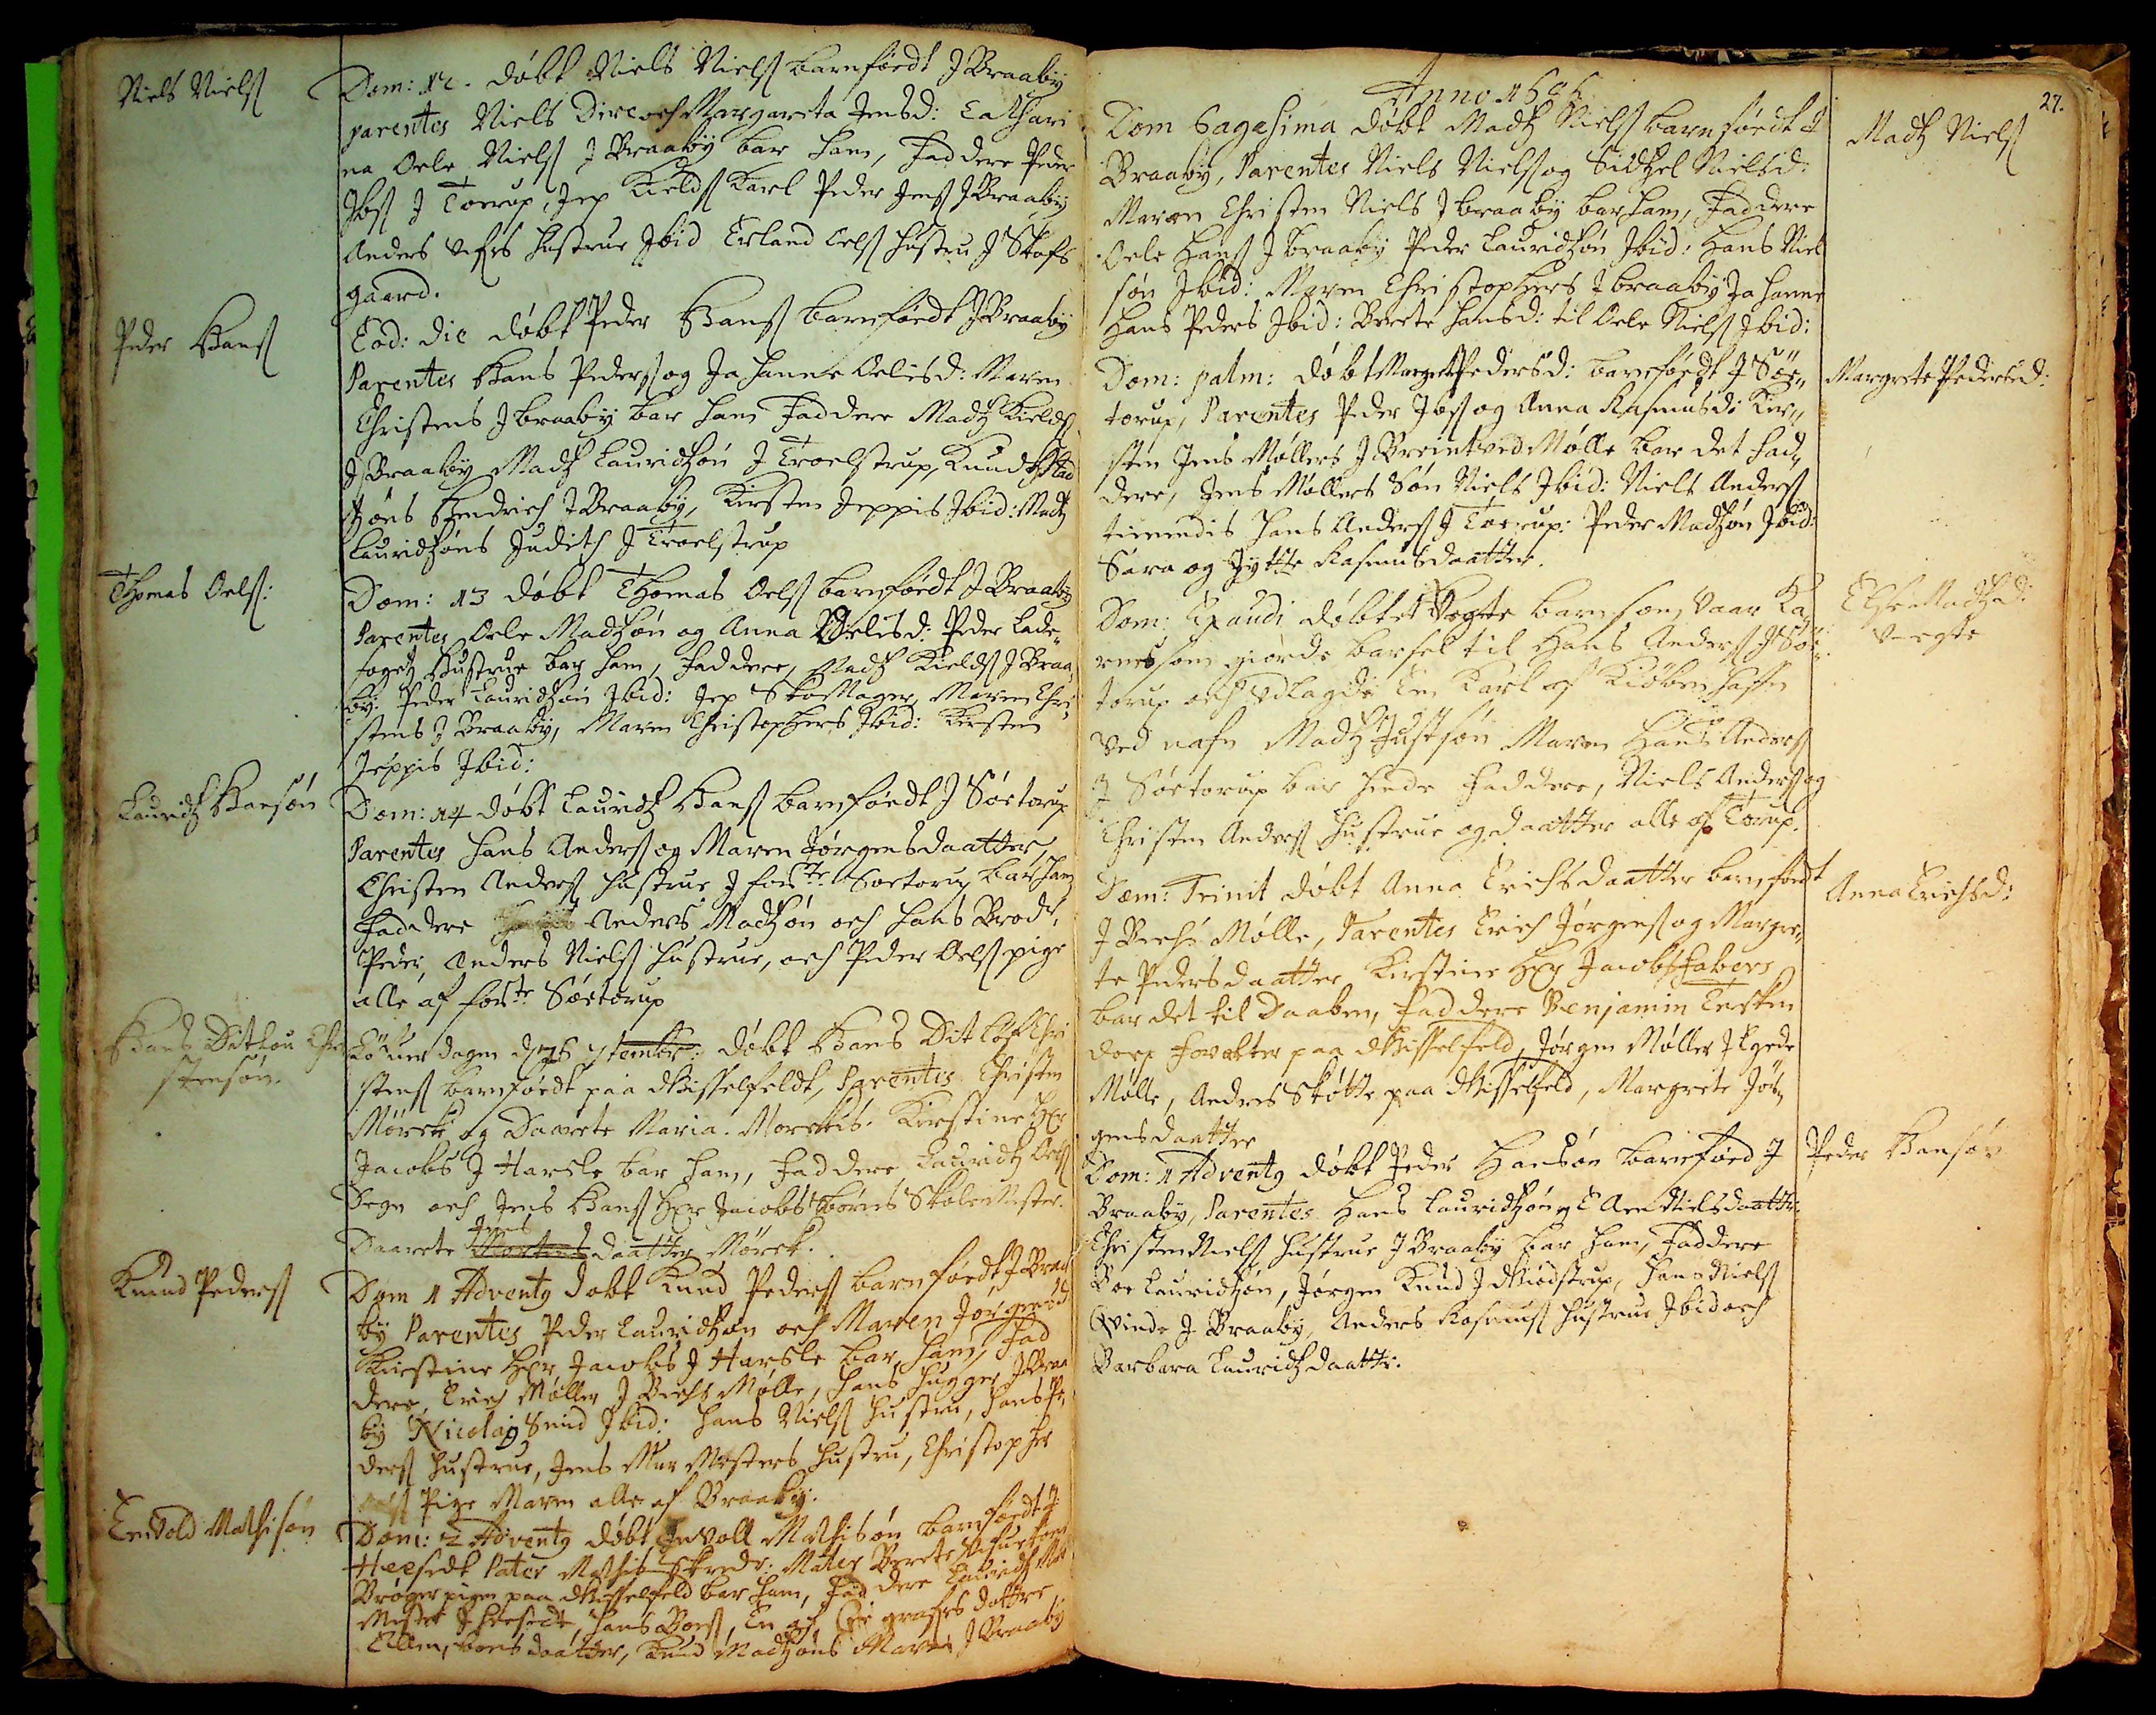Niels Niels
Dom: 12. døbt Niels Niels barnføedt J Braaby
Parentes Niels Dire och Margereta Jensd: Catheri
na Oele Niels J Braaby bar ham, Faddere Peder
Jbs J Toerup, Jep Kields karl Peder Jens J Braaby
Anders Vefis Hustrue Jbid, Erland Oels Hustru J Skofs
gaard.
Peder Hans
Eod: die døbt Peder Hanss barn føedt J Braaby
Parentes Hans Peders og Johanne Oelisd: Maren
Christens J Braaby, bar ham Faddere Mads Kields
J Braaby Mads Lauridsøn J Troelstrup, Knud Oele##
søns Hendrich J Braaby, Kirsten Jeppis Jbid: Mads
Lauridsøns Judith J Troelstrup.
Thomas Oels:
Dom: 13 døbt Thomas Oels barnføedt J Braaby
Parentes Oele Madsøn og Anna Oelisd: Peder Lade¬
fogetz Hustrue bar ham, Faddere Mads Kields J Braa¬
by: Peder Laurissøn Jbid: Jep SkoeMager, Maren Chri¬
stens J Braaby, Maren Christophers Jbid: Kirsten 
Jeppis Jbid: 
Lauridz Hansøn
Dom: 14 Lauridz Hans barn føedt J Søetorup,
Parentes Hans Anders og Maren Jørgensdaatter,
Christen Anders Hustrue J forte Søetorup bar ham.
Faddere: Hans Anders Madsøn och Hans Broder
Peder Anders Niels hustrue, och Peder Oels pige
alle af forte Søetorup.
Hans Ditleu Chri
stensøn
Løfuerdagen d 26 7tembr døbt Hans Ditløf Chri
stens barnføedt paa Gisselfeldt, Parentes: Christen
Mørck og Daarete Maria Mørckis Kirstine Hxr
Jacobs J Harsle bar ham, Faddere Laurids Oels
Degn och Jens Hans Hxr Jacobs børns SkoleMester,
Jens
Daarete Mortens daatter Mørck.
Knud Peders
Dom 1 Adventy døbt Knud Peders barn føedt J Braa
by, Parentes: Peder Lauridsøn och Maren Jørgensd:
Kirstene Hxr Jacobs J Harsle bar ham, Fad
dere, Erich Møller J Bechs Mølle, Hans Hugger J Braa
by, Nicolay Smid Jbid: Hans Niels Hustrue, Hans Pe¬
ders Hustrue, Jens MureMesters Hustrue, Christopher
Oels## Pige Maren alle af Braaby:
Envold Mathisøn
Dom: 2 Adventy døbt Envold Mathisøn barnføedt J
Heesedt, Pater Mathis Skreder: Mater Berete Vefur, ##
Brøger pige paa Gisselfeld bar ham, Faddere Laurids ##
Mester J Heesedt, Hans Boes, En af Cei grafzes dattre
Ellen Ceisdatter, Knud Madsøns Maren J Braaby.
Anno 1686.
Dom 6agesima døbt Madz Niels barn føedt J
Braaby, Parentes Niels Niels og Sidzel Nielsd:
Maren Christen Niels J Braaby bar ham, Faddere
Oele Hans J Braaby, Peder Lauridzøn Jbid. Hans Niel
søn Jbid: Maren Christophers J Braaby, Johanne
Hans Peders Jbid: Borete Hansd: til Oele Niels Jbid:
27.
Madz Niels
Dom: palm: døbt Margrete Pedersd: barnføedt J Søe¬
torup, Parentes Peder Jbs og Anna Rasmusd: Kir¬
sten Jens Møllers i Breintved Mølle bar det Fad¬
dere, Jens Møllers Søn Niels Jbid: Niels Anders
tienendis Hans Anders J Toerup: Peder Madsøn Jbid:
Sara og Jytte Rasmusdaatter. 
Margrete Pedersd:
Dom: Exaudi døbt et Uegte barn som vaar Ka¬
rens som giorde barsel til Hans Anders J Søe¬
torup och udlagde En Karl af Kiøbenhaffen
ved nafn Madz Justsøn, Maren Hans Anders
J Søetorup bar hinde Faddere, Niels Anders og
Christen Anders Hustrue og daatter alle af Toerup.
Else Madzd: 
U-egte
Dom: Trinit døbt Anna Erichsdatter barnføedt
J Bechs Mølle, Parentes Erich Jørgens og Margre¬
te Pedersdaatter, Kirsten Hxr Jacobs Fabers
bar det til Daaben, Faddere Benjamin Tesken##
dorp## forvalter paa Gisselfeld, Jørgen Møller J Egede
Mølle, Anders Skøtte paa Gisselfeld, Margrete Jør¬
gensdaatter.
Anna Erichsd:
Dom: 1 Adventy døbt Peder Hansøn barneføed J
Braaby, Parentes Hans Lauridsøn og Ellen Nielsdaatter,
Christen Niels Hustrue J Braaby bar ham, Faddere
Boe Lauridsøn, Jørgen Knud J Giødstrup, Hans Niels
Qvinde J Braaby, Anders Rasmus Hustrue Jbid. och
Barbara Lauridsdaatter.
Peder Hansøn
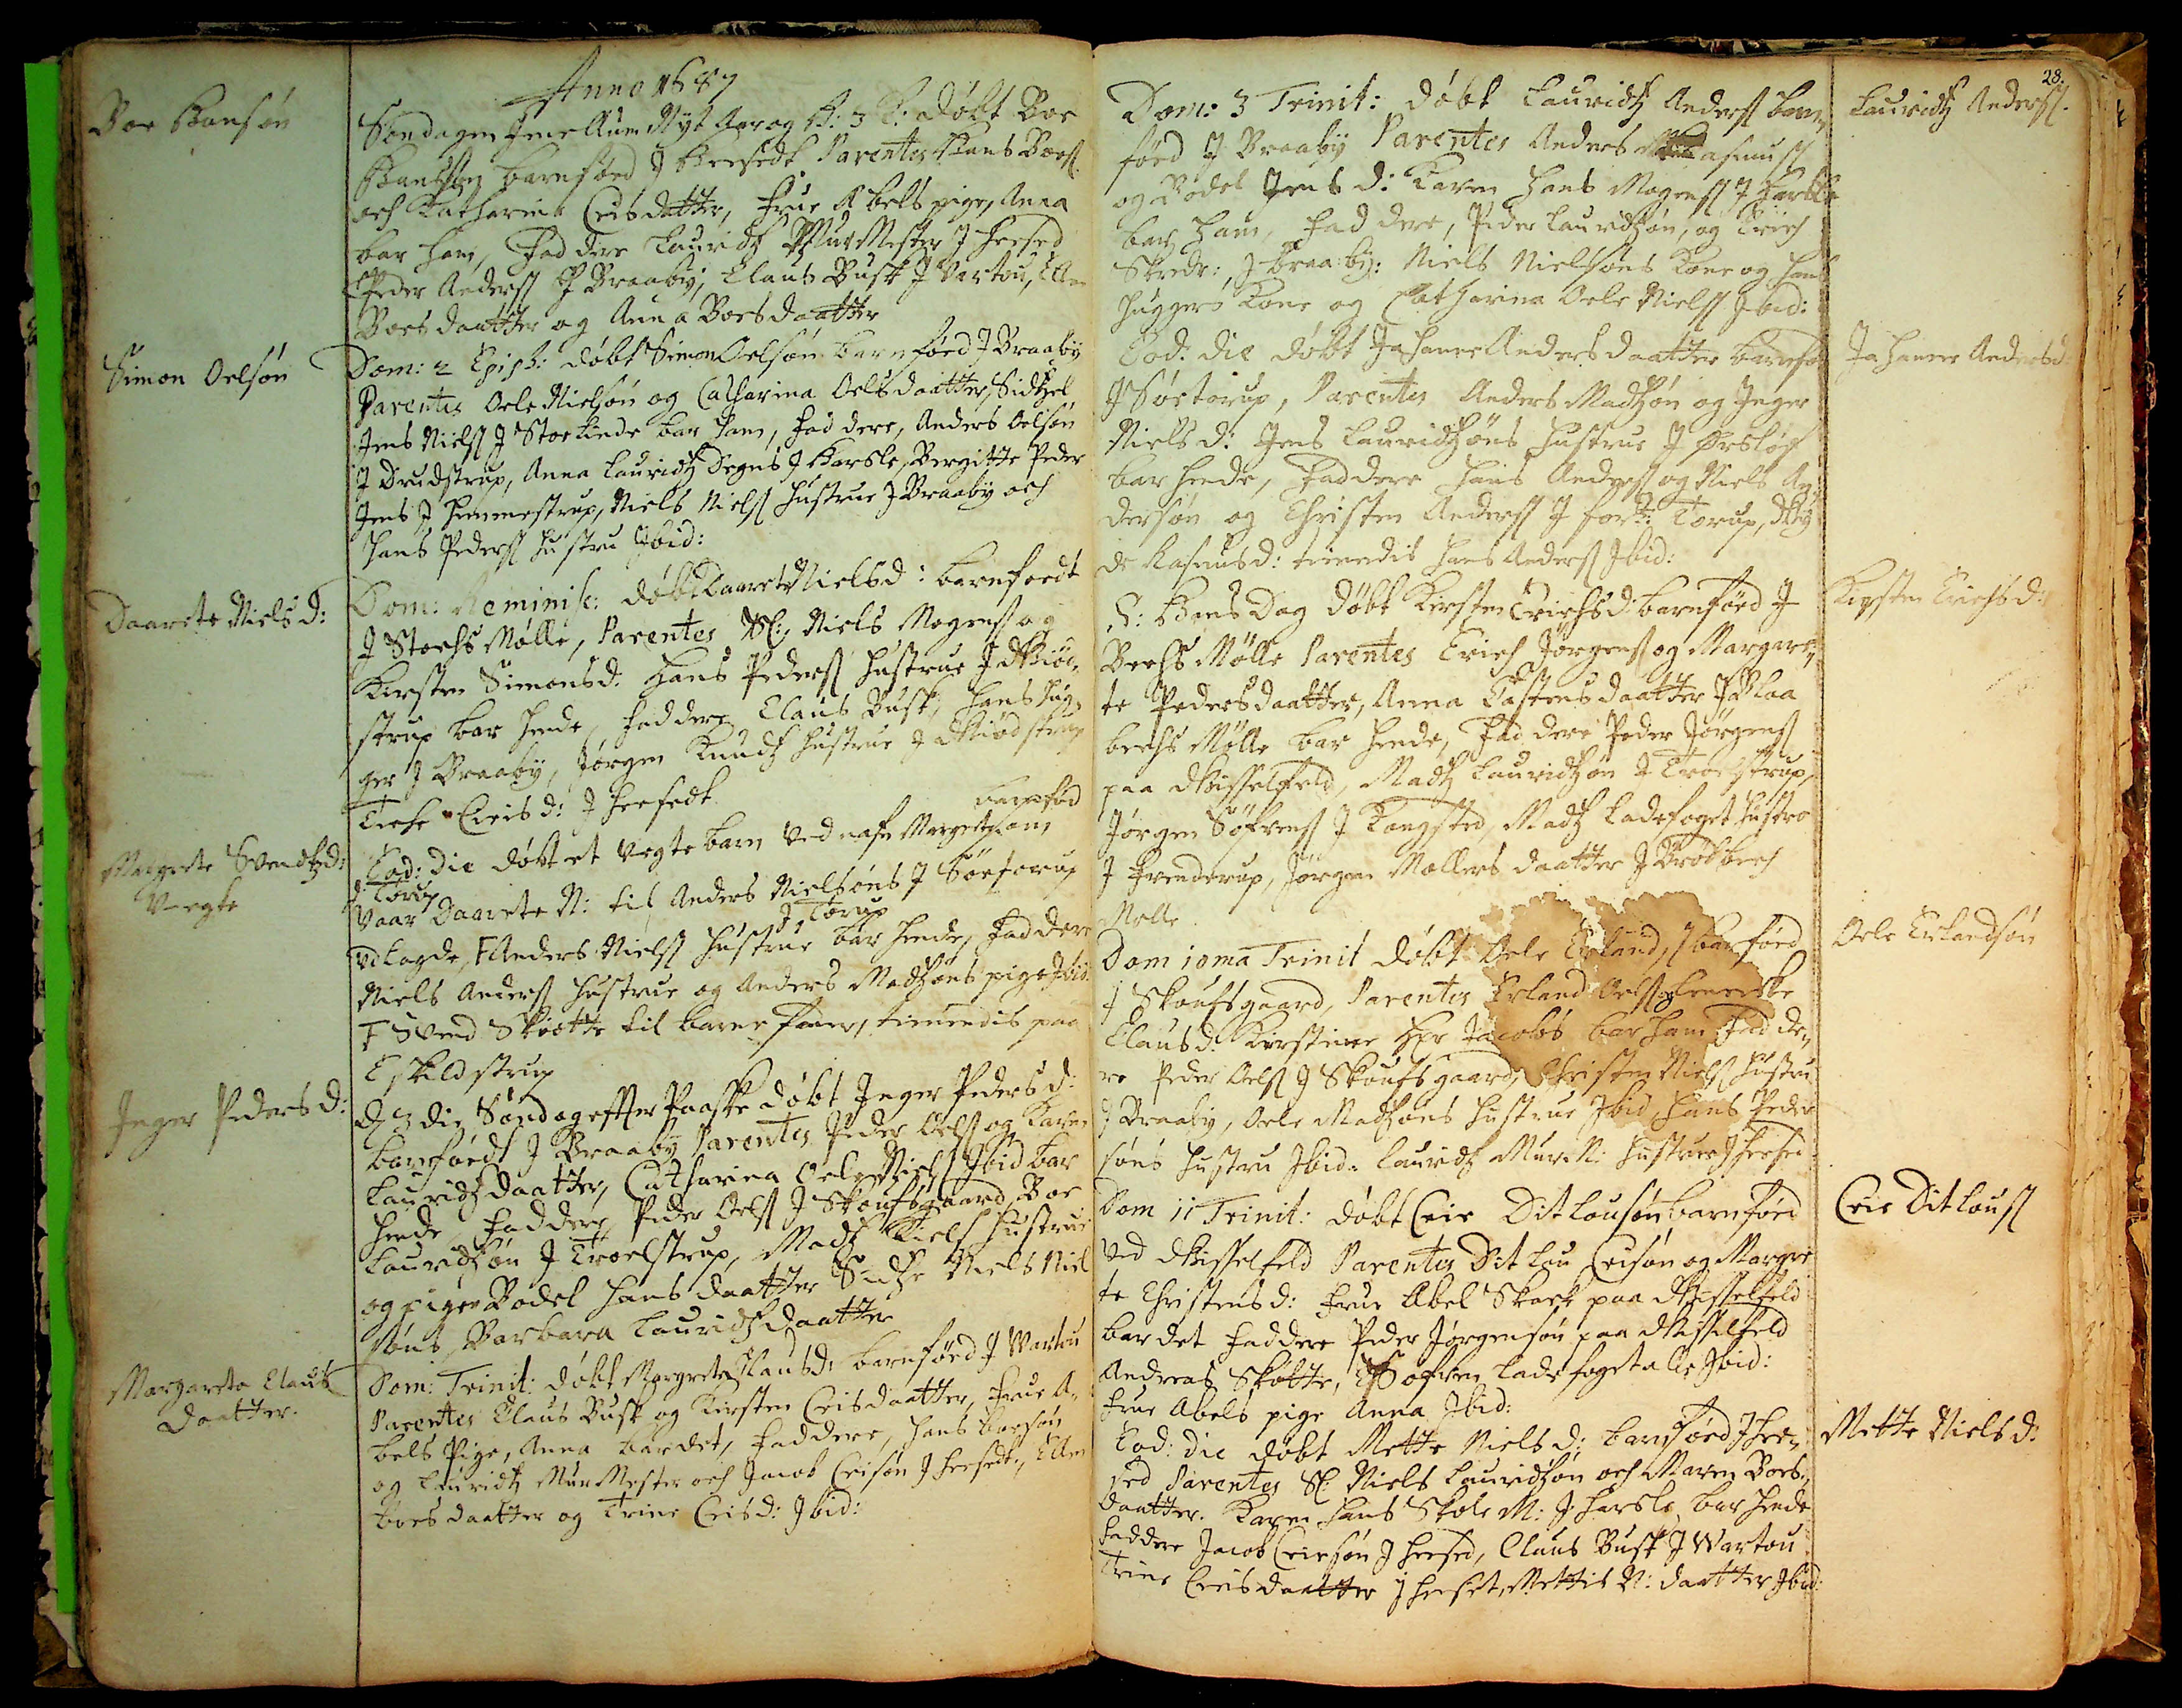Boe Hansøn 
Anno 1687 
Søndagen Imellum Nyt Aar og H: 3 K: døbt Boe 
Hansøn barnføed J Heesedt Parentes Hans Boes
och Katharine Ceisdatter, Frue Abels pige, Anna
bar ham, Faddere Laurids MurMester i Heesed
Peder Anders J Braaby; Claus Busk i Vartou, Ellen
Boes daatter og Anna Boes daatter.
Simon Oelsøn 
Dom: 2 Epiph døbt Simon Oelsøn barnføed J Braaby 
Parentes Oele Nielsøn og Catharina Oelsdaatter, Sidsel
Jens Niels J Stor Linde bar ham, Faddere, Anders Oelsøn
J Drudstrup, Anna Laurids Degns J Harsle, Bergitte Peder
Jens J Hemmestrup, Niels Niels Hustrue J Braaby och
Hans Peders Hustru Ibid:
Daarete Niels d: 
Dom: Reminisc: døbt Daarete Nielsd: barneføedt 
J Stoechs Mølle, Parentes Sl: Niels Mogens og
Kirsten Simonsd. Hans Peders Hustrue J Giød¬
strup bar hende, Faddere Claus Busk, Hans Hug¬
ger J Braaby, Jørgen Knuds Hustrue J Giødstrup
Tiehne## Ceis d: J Heesedt.
Margrete Svendsd:
Ueegte
barnefød
Eod: die døbt et Uegte barn ved nafn Margrete som
J Torup, 
vaar Daarete N: til Anders Nielsøn J Søetorup 
J Torup
udlagde F. Anders. Niels Hustrue bar hende, Fadder
Niels Anders Hustrue og Anders Madsøns pige Jbid
F. Svend Skiøtte til barne Fader, tienendis paa
Eskildstrup.
Jnger Peders d: 
D 3dig Søndag efter Paaske døbt Jnger Peders d: 
barneføedt J Braaby Parentes Peder Oels og Karen
Lauridsdaatter, Cathrina Oele Niels Jbid bar
hende, Faddere Peder Oels J Skoufsgaard, Boe
Lauridsøn J Troelstrup, Mads Kiels Hustrue
og piger Bodel Hans daatter, Sidse Niels Niel
søns, Barbara Lauridsdaatter.
Margrete Claus 
Daatter.
Dom: Trinit: døbt Margrete Clausd: barnføed J Vartou 
Parentes Claus Busk og Kirsten Ceisdaatter, Frue A¬
bels Pige, Anna bar det, Faddere, Hans Boesøn 
og Laurids, MurMester och Jacob Ceisøn J Heesedt, Ellen
Boes datter og Trine Ceisd: Jbid:
Dom: 3 Trinit: døbt Laurids Anders barn¬
føed J Braaby Parentes Anders Rasmus 
og Bodel Jensd: Karen Hans Mogens J Harsle
bar ham, Faddere, Peder Lauridsøn, og Erich
Skredr: J Braaby: Niels Nielsøns Kone og Hans
Huggers kone og Catherina Oele Niels Jbid:
28.
Lauridz Anders.
Eod: die døbt Johanne Andersdaatter barnefød 
J Søetorup, Parentes Anders Madsøn og Jnger
Nielsd:. Jens Lauridsøns Hustrue i Ørsløf
bar hende, Faddere Hans Anders og Niels An¬
dersøn og Christen Anders J Forte. Torup, Gy
de Rasmusd: tienndis Hans Anders Jbid:
Johanne Andersd: 
S: Hans Dag døbt Kirsten Erichsd: barnføed J
Bechs Mølle Parentes Erich Jørgens og Margare¬
te Peders daatter, Anna Castens daatter J Blaa
bechs Mølle bar hende, Faddere Peder Jørgens
paa Gisselfeld, Mads Lauridsøn J Troelstrup,
Jørgen Søfrens J Kongsted, Madz Ladefoget Hustrue
J Frenderup, Jørgen Møllers daatter J Brødbech
Mølle.
Kirsten Erichsd:
Dom 10.ma Trinit døbt Oele Erlands barnføed
J Skoufsgaard, Parentes Erland Oels og Gidske
Clausd: Kirsten Hxr. Jacobs bar ham, Fadde¬
re, Peder Oels J Skoufsgaard, Kirsten Niels Hustrue
J Braaby, Oele Madsøns Hustrue, Jbid. Hans Peder¬
søns Hustrue Jbid. Laurids Mur. M. Hustrue J Hessed.
Oele Erlandsøn
Dom 11 Trinit: døbt Ceis Ditlousøn barnføed 
ved Gisselfeld Parentes Ditlou Ceisøn og Marger
te Christensd: Frue Abel Skark paa Gisselfeld
bar det Faddere Peder Jørgensøn paa Gisselfeld
Andreas Skøtte, Søfren Ladefogete ## Jbid.
Frue Abels pige Anna Jbid.
Ceis Ditlous 
Eod: die døbt Mette Niels d: barnføed J Hee¬
sed Parentes Sl: Niels Lauridsøn och Maren Boes¬
daatter. Karen Hans SkoleM: J Harsle bar hinde
Faddere Jacob Ceissøn J Hersed, Claus Busk J Vartou:
Trine Ceisdaatter J Heeset, Mettis N: daatter Jbid:
Mette Niels d: